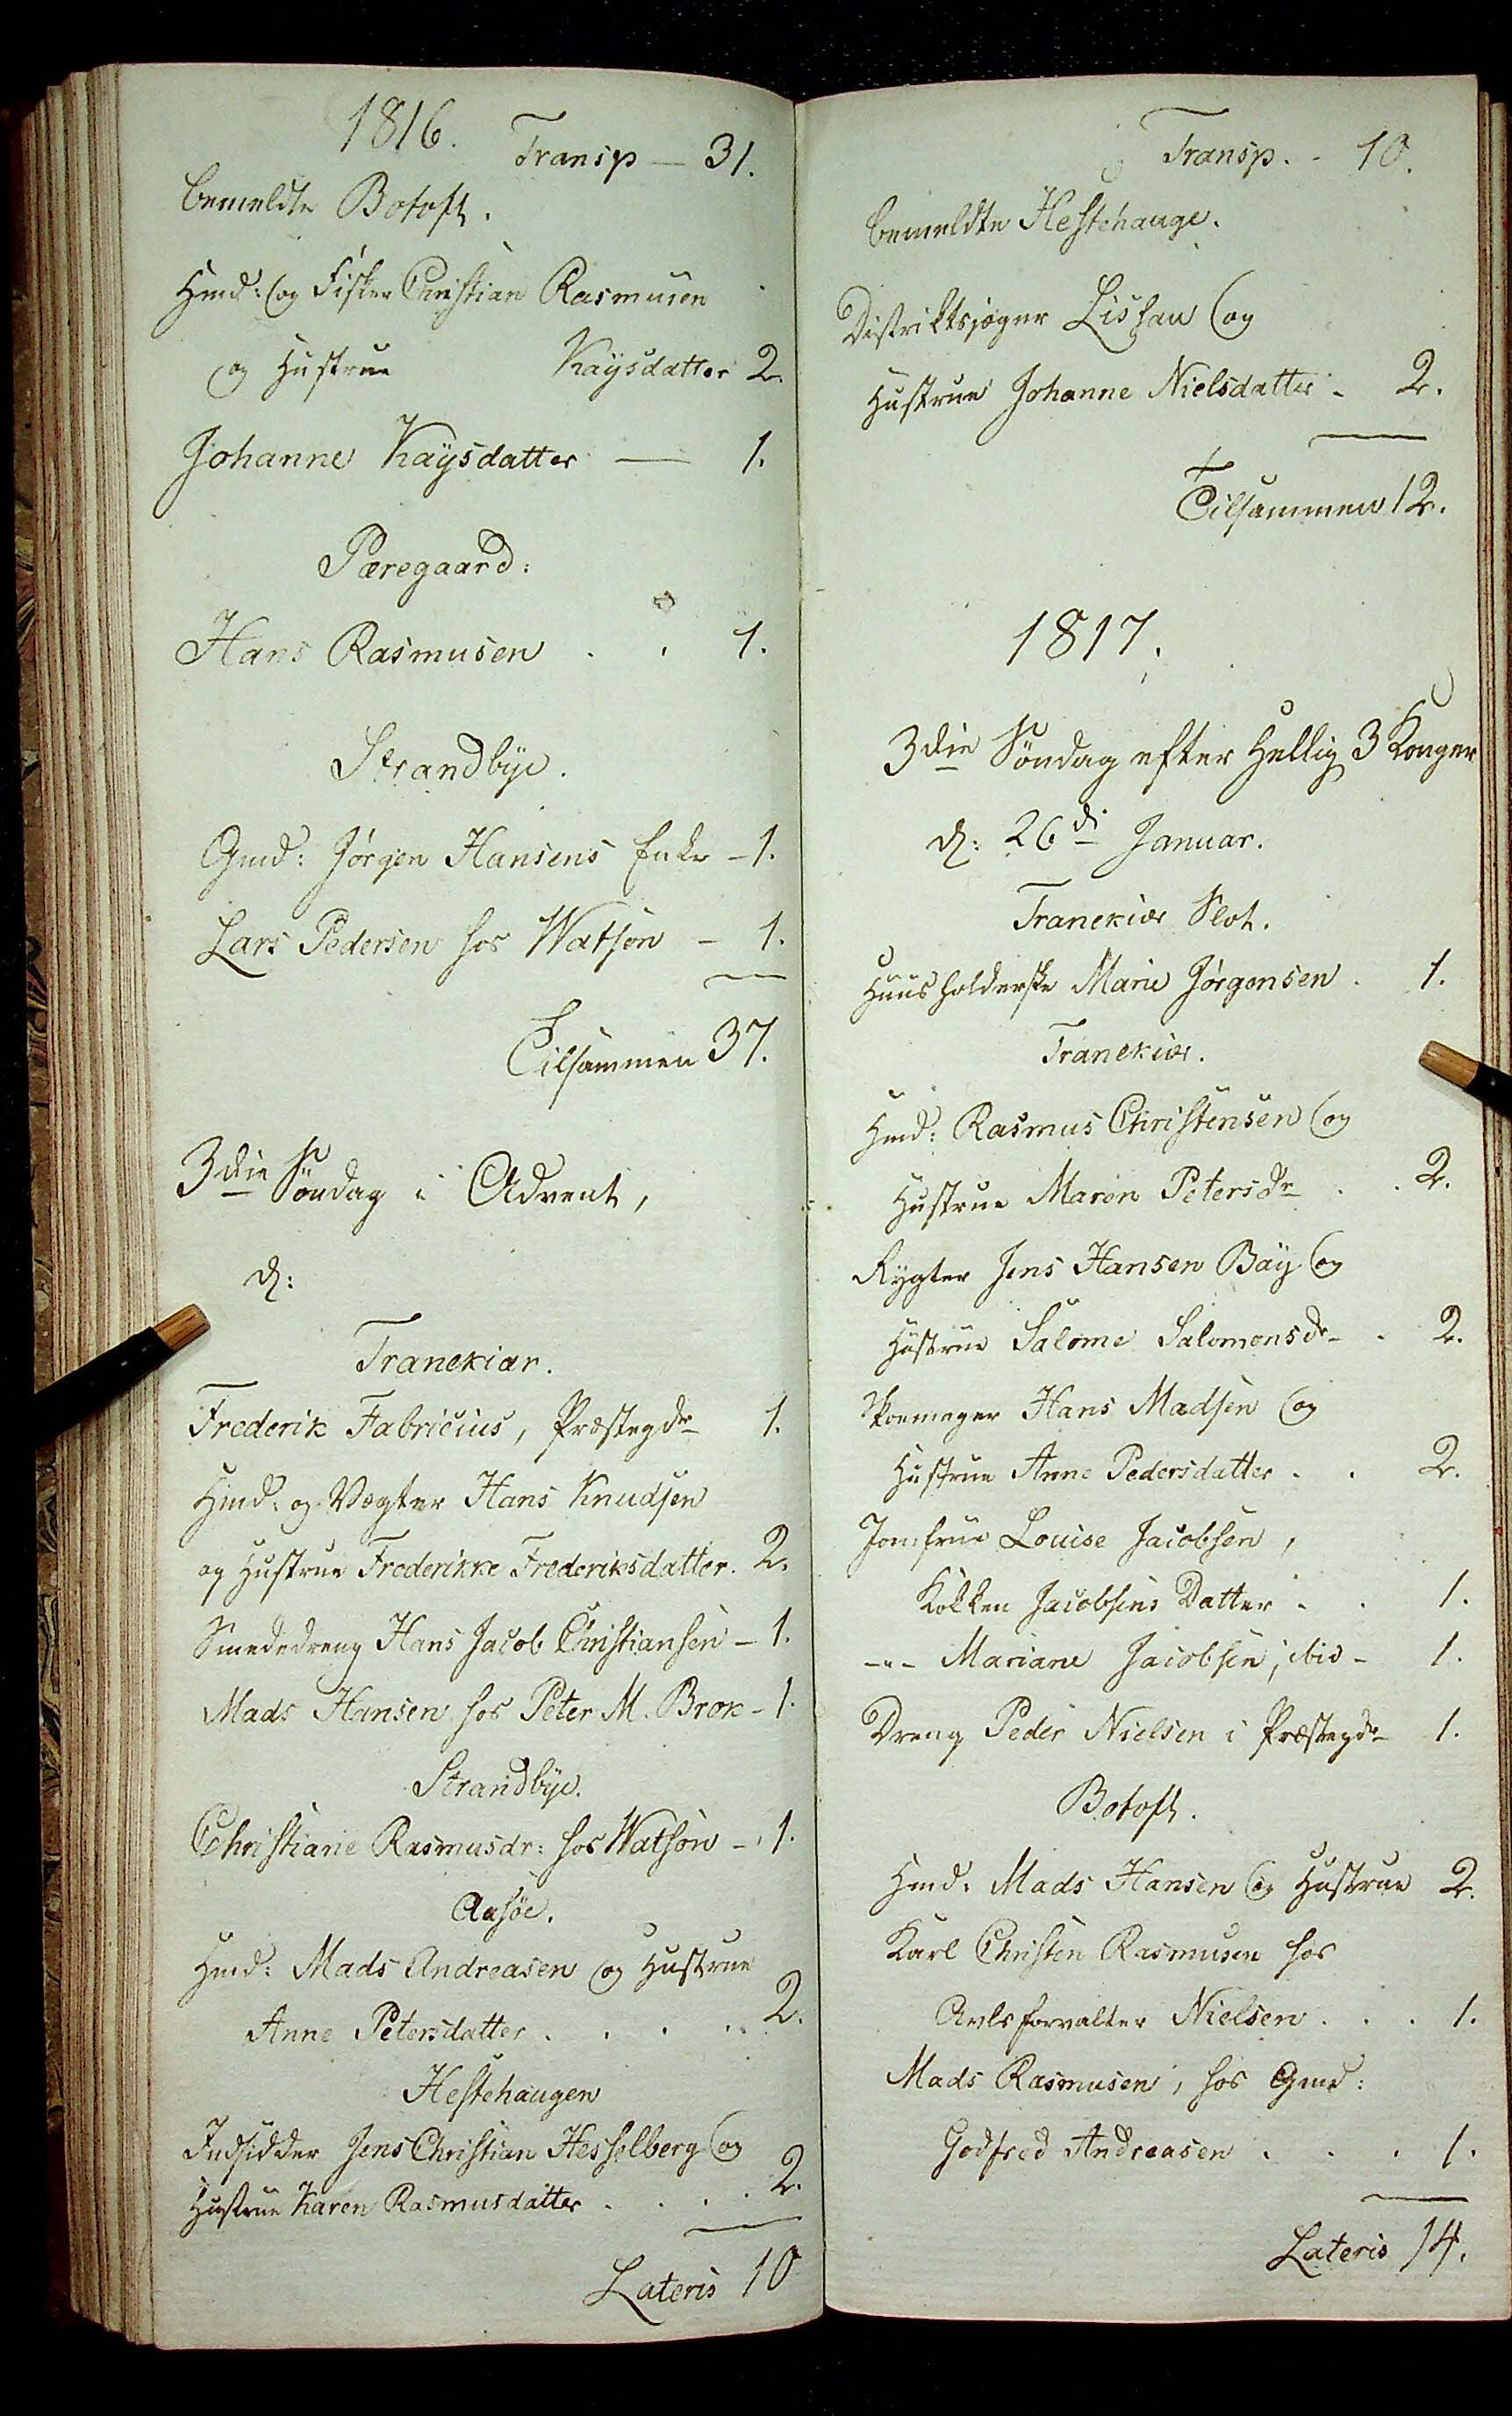1816. -
Transp 31.
bemeldte Botoft
Hmd: og Fisker Christian Rasmusen
og Hustrue Kaysdatter 2.
Johanne Kaysdatter - 1.
Pæregaard:
Hans Rasmusen . . 1.
Strandbye.
Gmd: Jørgen Hansens Enke - 1.
Lars Pedersen hos Watson - 1.
Tilsammen 37.
3die Søndag i Advent,
d:
Tranekiær.
Frederik Fabricius, Præstegdr 1.
Hind: og Vægter Hans Knudsen
og Hustrue Frederikke Frederichsdatter . 2.
Smededreng Hans Jacob Christiansen - 1.
Mads Hansen hos Peter M. Brok - 1.
Strandbye.
Christiane Rasmusdr: hos Waztson - . 1.
Aasøe.
Hmd: Mads Andreasen og Hustrue
Anne Petersdatter . . . . . 2.
Hestehaugen
Indsidder Jens Christian Hesselberg og
Hustrue Karen Rasmusdatter . . . . 2.
Lateris 10
Transp . . 10.
bemeldte Hestehauge.
Distriktsjæger Lissau og
Hustrue Johanne Nielsdatter - 2.
Tilsammen 12.
1817
3die Søndag efter Hellig 3 Konger
d: 26de Januar.
Tranekiær Slot.
Huusholderske Marie Jørgensen . 1.
Tranekiær.
Hmd: Rasmus Christensen og
Hustrue Maren Petersdr . . 2.
Rygter Jens Hansen Bay og
Hustrue Salome Salomonsdr . 2.
Skoemager Hans Madsen og
Hustrue Anne Pedersdatter . . 2.
Jomfrue Louise Jacobsen,
Kokken Jacobsens Datter . . 1.
-"- Mariane Jacobsen, ibid - 1.
Dreng Peder Nielsen i Præstegdr 1.
Botoft.
Hmd: Mads Hansen og Hustrue 2.
Karl Christen Rasmusen hos
Avlsforvalter Nielsen . . . 1.
Mads Rasmusen, hos Gmd:
Godfred Andreasen . . . 1.
Lateris 14.
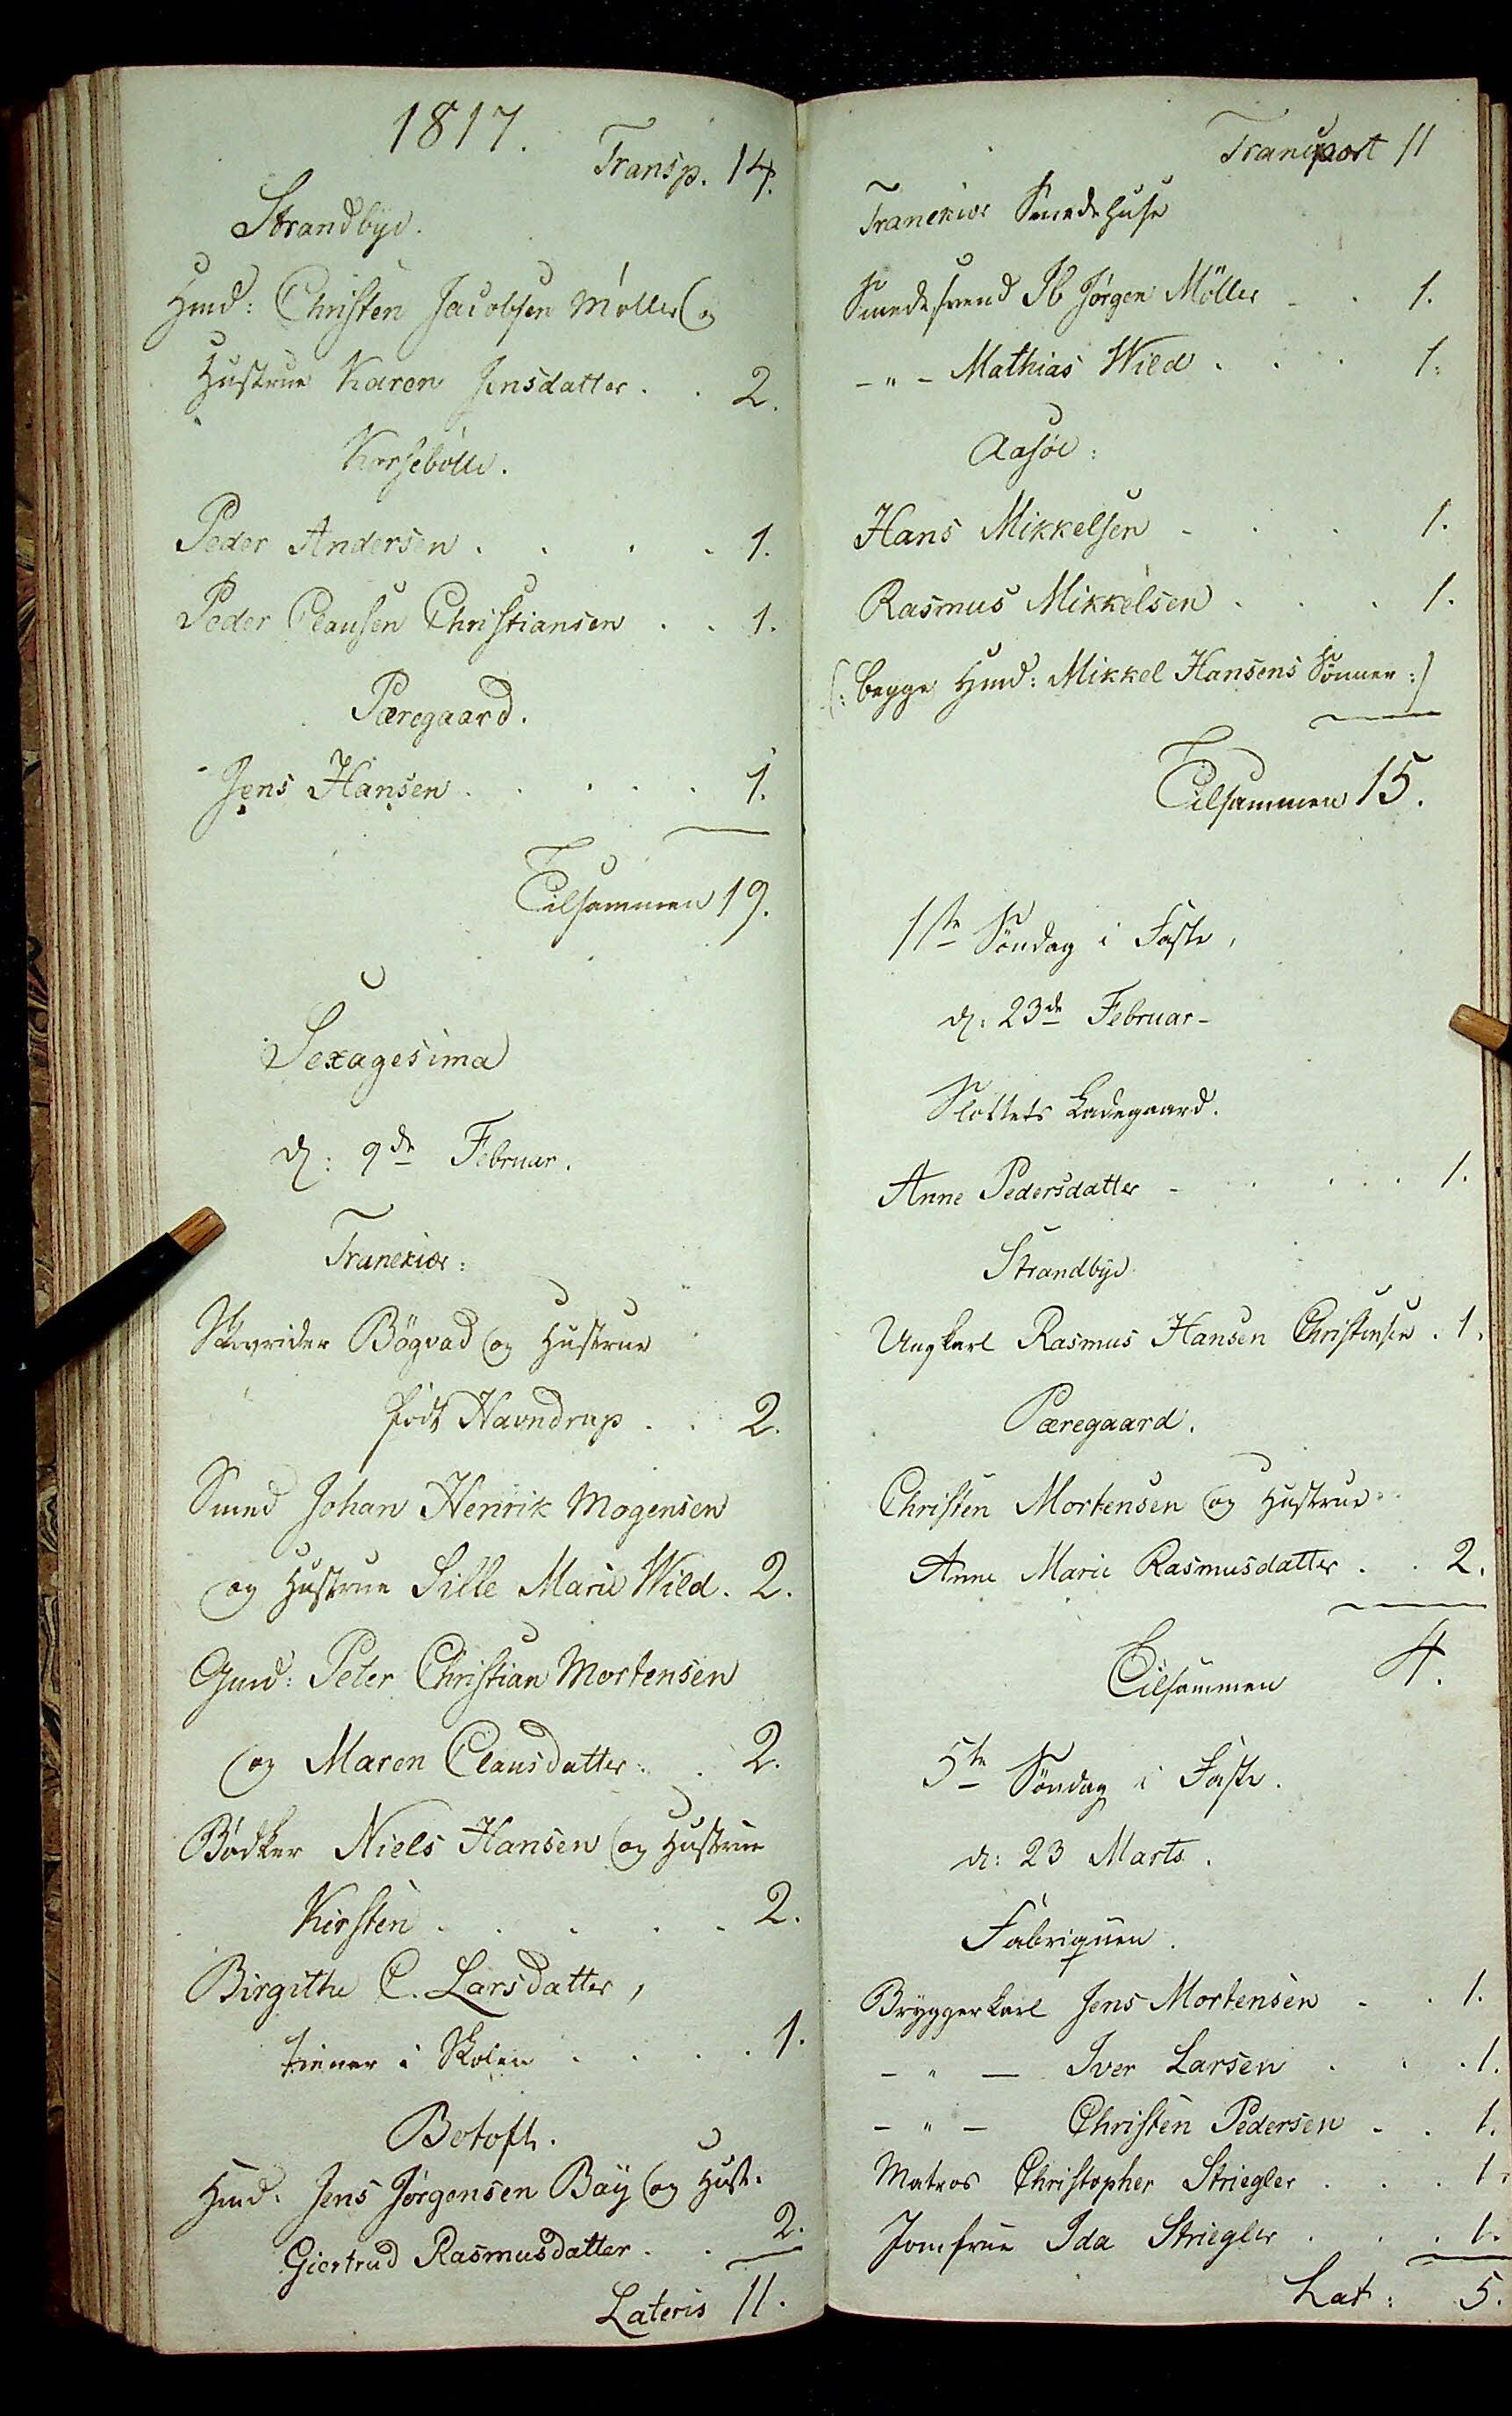1817.
Transp. 14.
Strandbye.
Hmd: Christen Jacobsen Møller og
Hustrue Karen Jensdatter . . 2.
Korsebølle.
Peder Andersen. . . . 1.
Peder Clausen Christiansen . . 1.
Pæregaard.
Jens Hansen . . . . . 1.
Tilsammen 19.
Sexagesima
d: 9de Februar.
Tranekiær:
Skovrider Bøgvad og Hustrue
født Havndrup . .2.
Smed Johan Henrik Mogensen
og Hustrue Sille Mari Wild . 2.
Gmd: Peter Christian Mortensen
og Maren Clausdatter . . 2.
Bødker Niels Hansen og Hustrue
Kirsten . . . . . 2.
Birgitte C. Larsdatter,
tiener i Skolen . . . . 1.
Betoft.
Hmd. Jens Jørgensen Bay og Hust:
Giertrud Rasmusdatter . . 2.
Lateris 11
Transport 11
Tranekiær Smedehuse
Smedesvend Ib Jørgen Møller - . 1.
-"- Mathias Wild . . . 1.
Aasøe:
Hans Mikkelsen . . .
Rasmus Mikkelsen . . . 1.
begge Hmd: Mikkel Hansens Sønner :/
Tilsammen 15.
1ste Søndag i Faste,
d: 23de Februar-
Slottets Ladegaard.
Anne Pedersdatter - . . . . 1.
Strandbye
Ungkarl Rasmus Hansen Christensen . 1.
Pæregaard.
Christen Mortensen og Hustrue.
Anne Marie Rasmusdatter . . 2.
Tilsammen 4.
5te Søndag i Faste.
d: 23 Marts.
Fabriquen
Bryggerkarl Jens Mortensen . . 1.
-"- Iver Larsen . . . 1.
-"- Christen Pedersen . . 1.
Matros Christopher Striegler . . . 1.
Jomfrue Ida Striegler . . . 1.
Lat: 5.